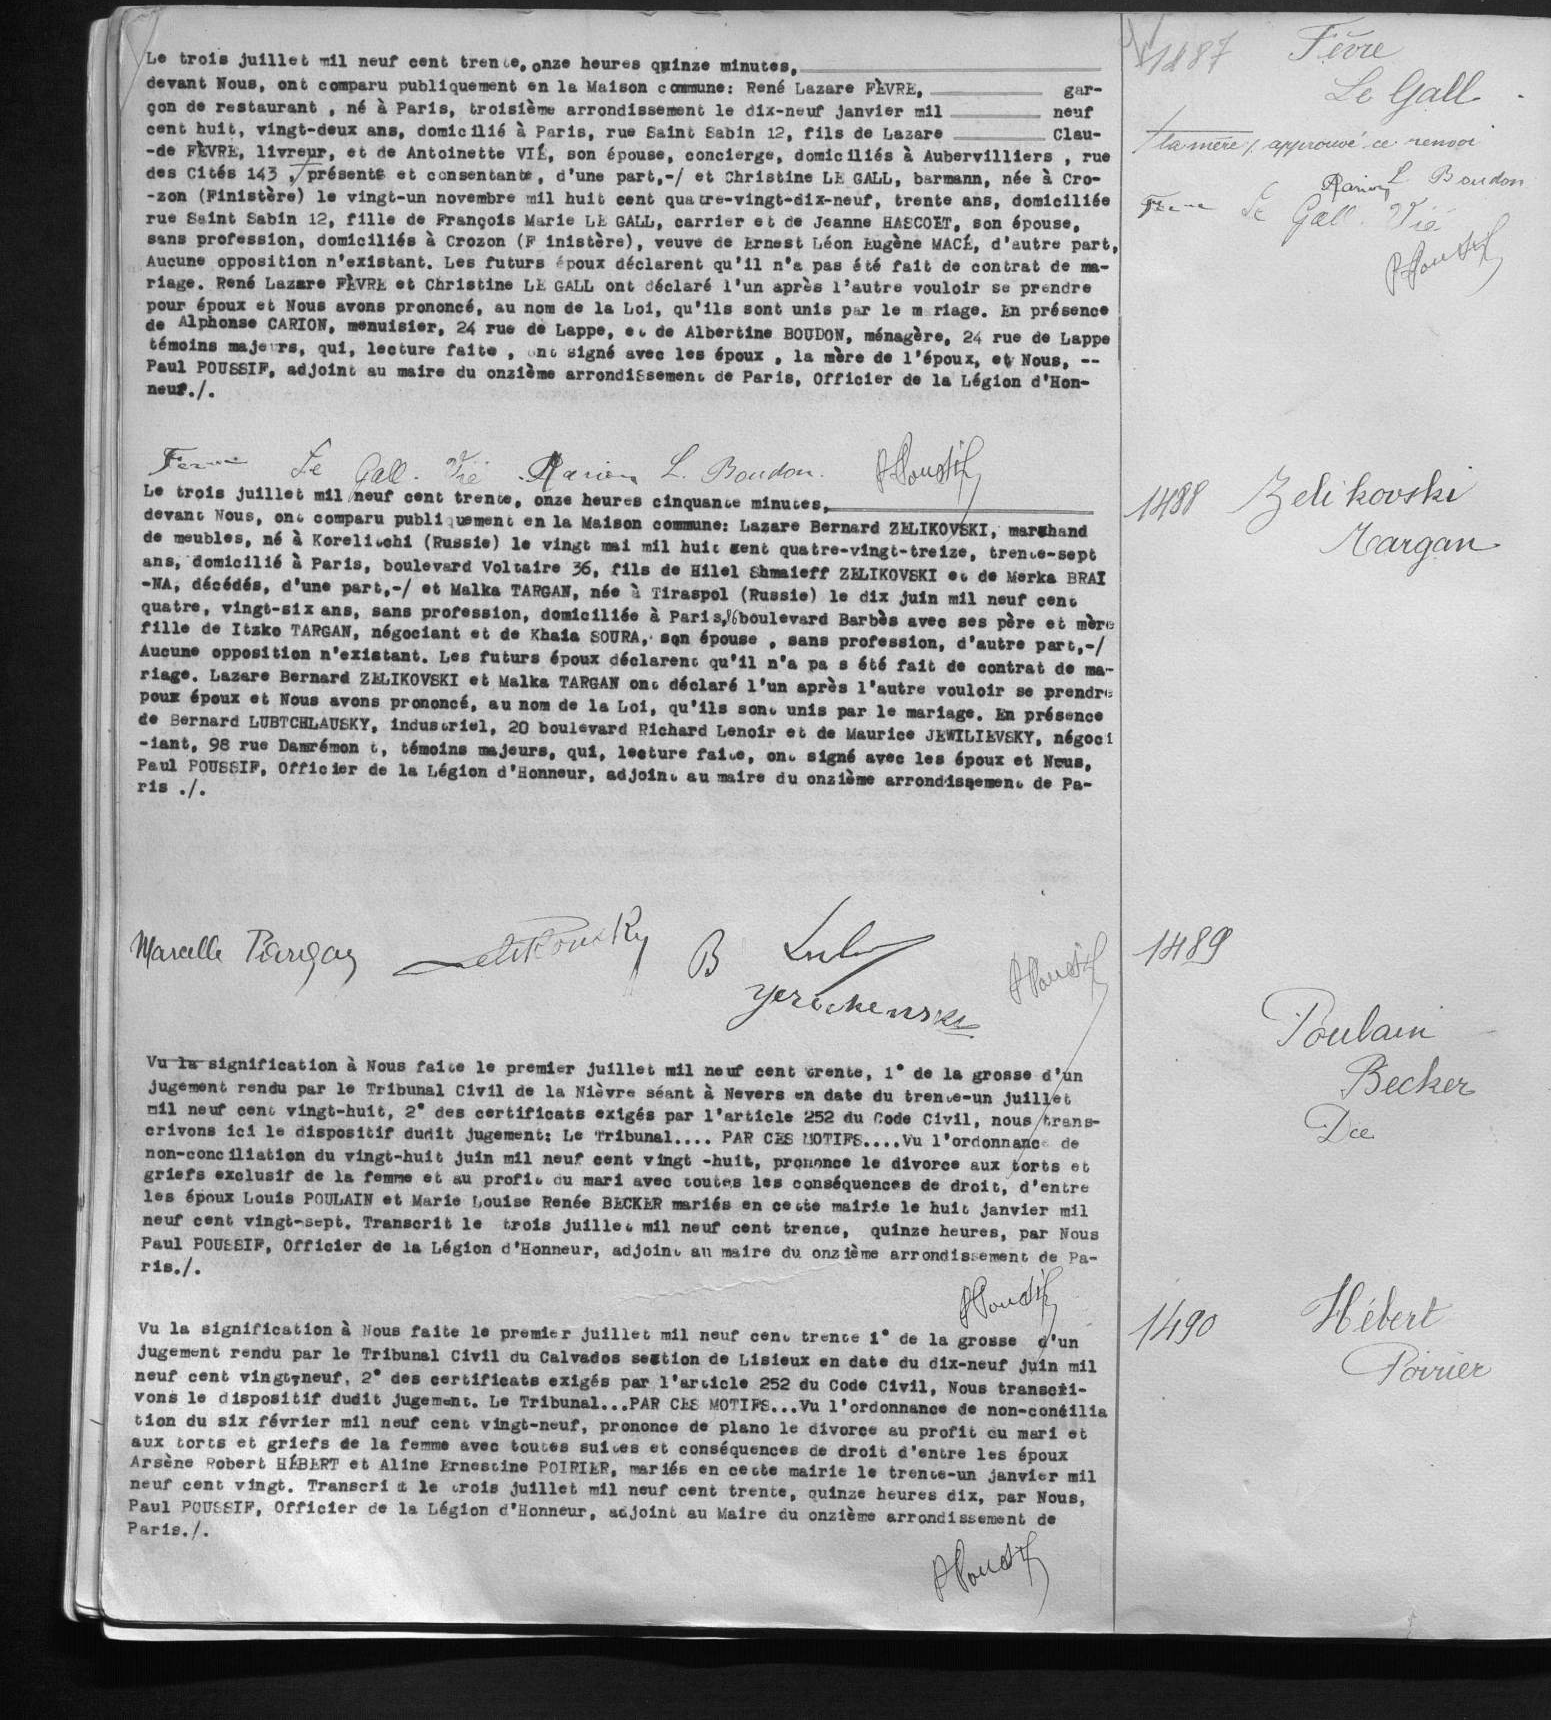Le trois juillet mil neuf cent trente, onze heures quinze minutes ,-
devant Nous, ont comparu publiquement en la Maison commune: René Lazare FEVRE ,-, gar-
çon de restaurante, né à Paris, troisième arrondissement le dix-neuf janvier mil -neuf
cent huit, vingt-deux ans, domicilié à Paris, rue Saint Sabin 12, fils de Lazare -Clau-
-de FEVRE, livreur, et de Antoinette VIE, son épouse, concierge, domiciliés à Aubervilliers, rue
des Cités 143, présente et consentante, d'une part ,-/ et Christine LE GALL, barmann, née à Cro-
-zon (Finistère) le vingt-un novembre mil huit cent quatre-vingt-dix-neuf, trente ans, domiciliée
rue Saint Sabin 12, fille de François Marie LE GALL, carrier et de Jeanne HASCOET, son épouse,
sans profession, domiciliés à Crozon (F inistère), veuve de Ernest Léon Eugène MACE, D'AUTRE PART,
Aucune opposition n'existante. Les futurs époux déclarent qu'il n'a pas été fait de contrat de ma-
riage. René Lazare FEVRE et Christine LE GALL ont déclaré l'un après l'autre vouloir se prendre
pour époux et Nous avons prononcé au nom de la Loi, qu'ils sont unis par le mariage. En présence
de Alphonse CARION, menuisier, 24 rue de Lappe, e de Albertine BOUDON, ménagère, 24 rue de Lappe
témoins majeurs, qui, lecture faite, ont signé avec les époux, la mère de l'époux, et Nous, -
Paul POUSSIF, adjoint au maire du onzième arrondissemen de Paris, Officier de la Légion d'Hon-
neuf ./.
Le trois juillet mil neuf cent trente, onze heures cinquante minutes, -
devant Nous, ont comparu publiquement en la Maison commune: Lazare Bernard ZELIKOVSK, marchand
de meubles, né à Koreliechi (Russie) le vingt mai mil huit cent quatre-vingt-treize, trente-sept
ans, domicilié à Paris, boulevard Voltaire 36, fils de Hilel Schmieff ZELIKOVSKI et de Merka BRAI
-NA, décédés, d'une part ,-/ et Malka TARGAN, née à Tiraspol (Russie) le dix juin mil neuf cent
quatre, vingt-six ans, sans profession, domiciliée à Paris, 86 boulevard Barbès avec ses père et mère
fille de Itzko TARGAN, négociant et de Khaia SOURA, son épouse, sans profession, d'autre part ,-/
Aucune opposition n'existant. Les futurs époux déclarent qu'il n'a pas été fait de contrat de ma-
riage. Lazare Bernard ZELIKOVSKI et Malka TARGAN ont déclaré l'un après l'autre vouloir se prendre
pour époux et Nous avons prononcé, au nom de la loi, qu'ils sont unis par le mariage. En présence
de Bernard LUBTCHLAUSKY, industriel, 20 boulevard Richard Lenoir et de Maurice JEWLIEVSKY, négoci
-iant, 98 rue Damrémont, témoins majeurs, qui, lecture faite, ont signé avec les époux et Nous,
Paul POUSSIF, Officier de la Légion d'Honneur, adjoint au maire du onzième arrondissement de Pa-
ris ./.
Vu la signification à Nous faite le premier juillet mil neuf cent trente, 1° de la grosse d'un
jugement rendu par le Tribunal Civil de la Nièvre séant à Nevers en date du trente-un juillet
mil neuf cent vingt-huit, 2° des certificats exigés par l'article 252 du Code Civil, nous trans-
crivons ici le dispositif dudit jugement: Le Tribunal... PAR CES MOTIFS... Vu l'ordonnance de
non-conciliation du vingt-huit juin mil neuf cent vingt-huit, prononce le divorce aux torts et
griefs exclusif de la femme et au profit du mari avec toutes les conséquences de droit, d'entre
les époux Louis POULAIN et Marie Louise Renée BECKER mariés en cette mairie le huit janvier mil
neuf cent vingt-sept. Transcrit le trois juillet mil neuf cent trente, quinze heures, par Nous
Paul POUSSIF, Officier de la Légion d'Honneur, adjoint au maire du onzième arrondissement de Pa-
ris ./.
Vu la signification à Nous faite le premier juillet mil neuf cent trente 1° de la grosse d'un
jugement rendu par le Tribunal Civil du Calvados section de Lisieux en date du dix-neuf juin mil
neuf cent vingt-neuf, 2° des certificats exigés par l'article 252 du Code Civil, Nous transcri-
vons le dispositif dudit jugement. Le Tribunal... PAR CES MOTIFS... Vu l'ordonnance de non-concilia
tion du six février mil neuf cent vingt-neuf, prononce de plano le divorce au profit du mari et
aux torts et griefs de la femme avec toutes suites et conséquences de droit d'entre les époux
Arsène Robert HEBERT et Aline Ernestine POIRIER, mariés en cette mairie le trente-un janvier mil
neuf cent vingt. Transcrit le trois juillet mil neuf cent trente, quinze heures dix, par Nous,
Paul POUSSIF, Officier de la Légion d'Honneur, adjoint au Maire du onzième arrondissement de
Paris ./.
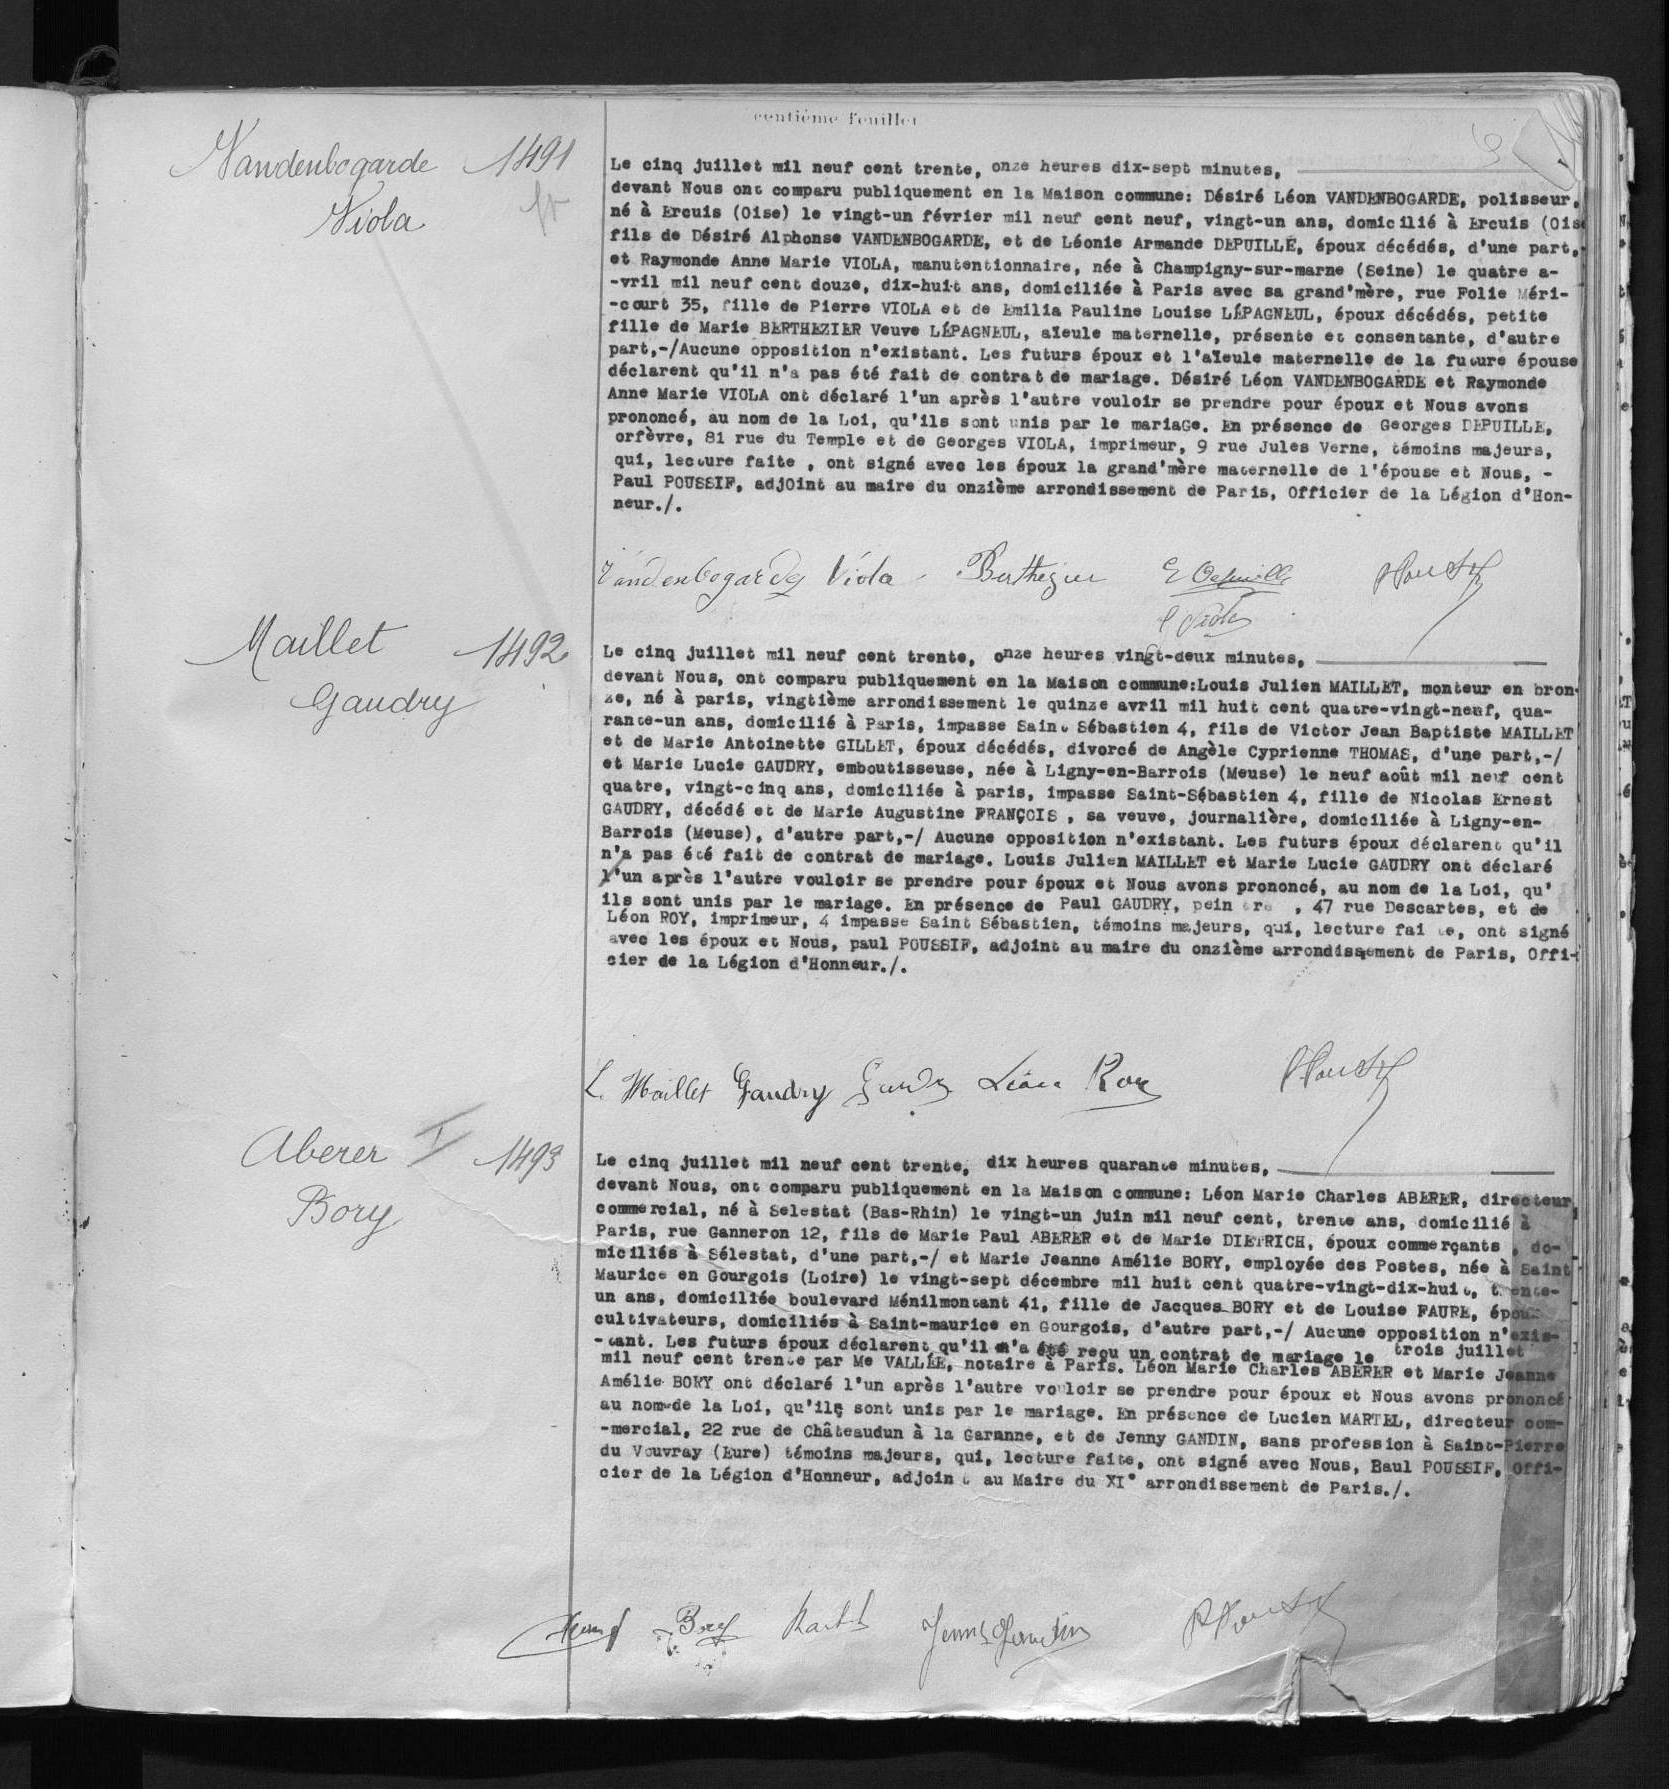Le cinq juillet mil neuf cent trente, onze heures dix-sept minutes ,-
devant Nous ont comparu publiquement en la Maison commune: Désiré Léon VANDENBOGARDE, polisseur,
né à Ercuis (Oise) le vingt-un février mil neuf cent neuf, vingt-un ans, domicilié à Ercuis (Oise
fils de Désiré Alphonse VANDENBOGARDE, et de Léonie Armande DEPUILLE, époux décédés, d'une part ,-
et Raymonde Anne Marie VIOLA, manutentionnaire, née à Champigny-sur-marne (Seine) le quatre a-
-vril mil neuf cent douze, dix-huit ans, domiciliée à Paris avec sa grand'mère, rue Folie Méri-
-court 35, fille de Pierre VIOLA et de Emilia Pauline Louise EPAGNEUL, époux décédés, petite
fille de Marie BERTHEZIER Veuve LEPAGNEUL, aïeule maternelle, présente et consentante, d'autre
part ,-/ Aucune opposition n'existant. Les futurs époux et l'aïeule maternelle de la future épouse
déclarent qu'il n'a pas été fait de contrat de mariage. Désiré Léon VANDENBOGARDE et Raumonde
Anne Marie VIOLA ont déclaré l'un après l'autre vouloir se prendre pour époux et Nous avons
prononcé, au nom de la Loi, qu'ils sont unis par le mariage. En présence de Georges DEPUILLE,
orfèvre, 81 rue du Temple et de Georges VIOLA, imprimeur, 9 rue Jules Verne, témoins majeurs,
qui, lecture faite, ont signé avec les époux la grand'mère maternelle de l'épouse de Nous,-
Paul POUSSIF, adj0int au maire du onzième arrondissement de Paris, Officier de la Légion d'Hon-
neur ./.
Le cinq juillet mil neuf cent trente, onze heures vingt-deux minutes ,-
devant Nous, ont comparu publiquement en la Maison commune: Louis Julien MAILLET, monteur en bron-
ze, né à paris, vingtième arrondissement le quinze avril mil huit cent quatre-vingt-neuf, qua-
rante-un ans, domicilié à Paris, impasse Sain Sébastien 4, fils de Victor Jean Baptiste MAILLET
et de Marie Antoinette GILLET, époux décédés, divorcé de Angèle Cyprienne THOMAS, d'une part ,-/
et Marie Lucie GAUDRY, emboutisseuse, née à Ligny-en-Barrois (Meuse) le neuf août mil neuf cent
quatre, vingt-cinq ans, domiciliée à paris, impasse Saint-Sébastien 4, fille de Nicolas Ernest
GAUDRY, décédé et de Marie Augustine FRANCOIS, sa veuve, journalière, domiciliée à Ligny-en-
Barrois (Meuse), d'autre part ,-/ Aucune opposition n'existant. Les futurs époux déclarent qu'il
n'a pas été fait de contrat de mariage. Louis Julien MAILLET et Marie Lucie GAUDRY ont déclaré
l'un après l'autre vouloir se prendre pour époux et Nous avons prononcé, au nom de la Loi, qu'
ils sont unis par le mariage. En présence de Paul GAUDRY, pein re, 47 rue Descartes, et de
Léon ROY, imprimeur, 4 impasse Saint Sébastien, témoins majeurs, qui, lecture fai e, ont signé
avec les époux et Nous, paul POUSSIF, adjoint au maire du onzième arrondissement de Paris, Offi)
cier de la Légion d'Honneur ./.
Le cinq juillet mil neuf cent trente, dix heures quarante minutes ,-
devant Nous, ont comparu publiquement en la Maison commune: Léon Marie Charles ABERER, directeur
commercial, né à Selestat (Bas-Rhin) le vingt-un juin mil neuf cent, trente ans, domicilié à
Paris, rue Ganneron 12, fils de Marie Paul ABERER et de Marie DIETRICH, époux commerçants, do-
miciliés à Sélestat, d'une part ,-/ et Marie Jeanne Amélie BORY, employée des Postes, née à Saint
Maurice en Gourgois (Loire) le vingt-sept décembre mil huit cent quatre-vingt-dix-huit, trente-
un ans, domiciliée boulevard Ménilmontant 41, fille de Jacques BORY et de Louis FAURE, épous
cultivateurs, domiciliés à Saint-maurice en Gourgois, d'autre part ,-/ AUcune opposition n'exis-
-tant. Les futurs époux déclarent qu'il * n'a été reçu un contrat de mariage le trois juille
mil neuf cent trente par Me VALLEE, notaire à Paris. Léon Marie Charles ABERER et Marie Jeann
Amélie BORY ont déclaré l'un après l'autre vouloir se prendre pour époux et Nous avons prononcé
au nom de la Loi, qu'ils sont unis par le mariage. En présence de Lucien MARTEL, directeur com-
-mercial, 22 rue de Châteaudun à la Garanne, et de Jenny GANDIN, sans profession à Saint-Pierre
du Vouvray (Eure) témoins majeurs, qui, lecture faite, ont signé avec Nous, Baul POUSSIF, Offi-
cier de la Légion d'Honneur, adjoin au Maire du XI ° arrondissement de Paris ./.